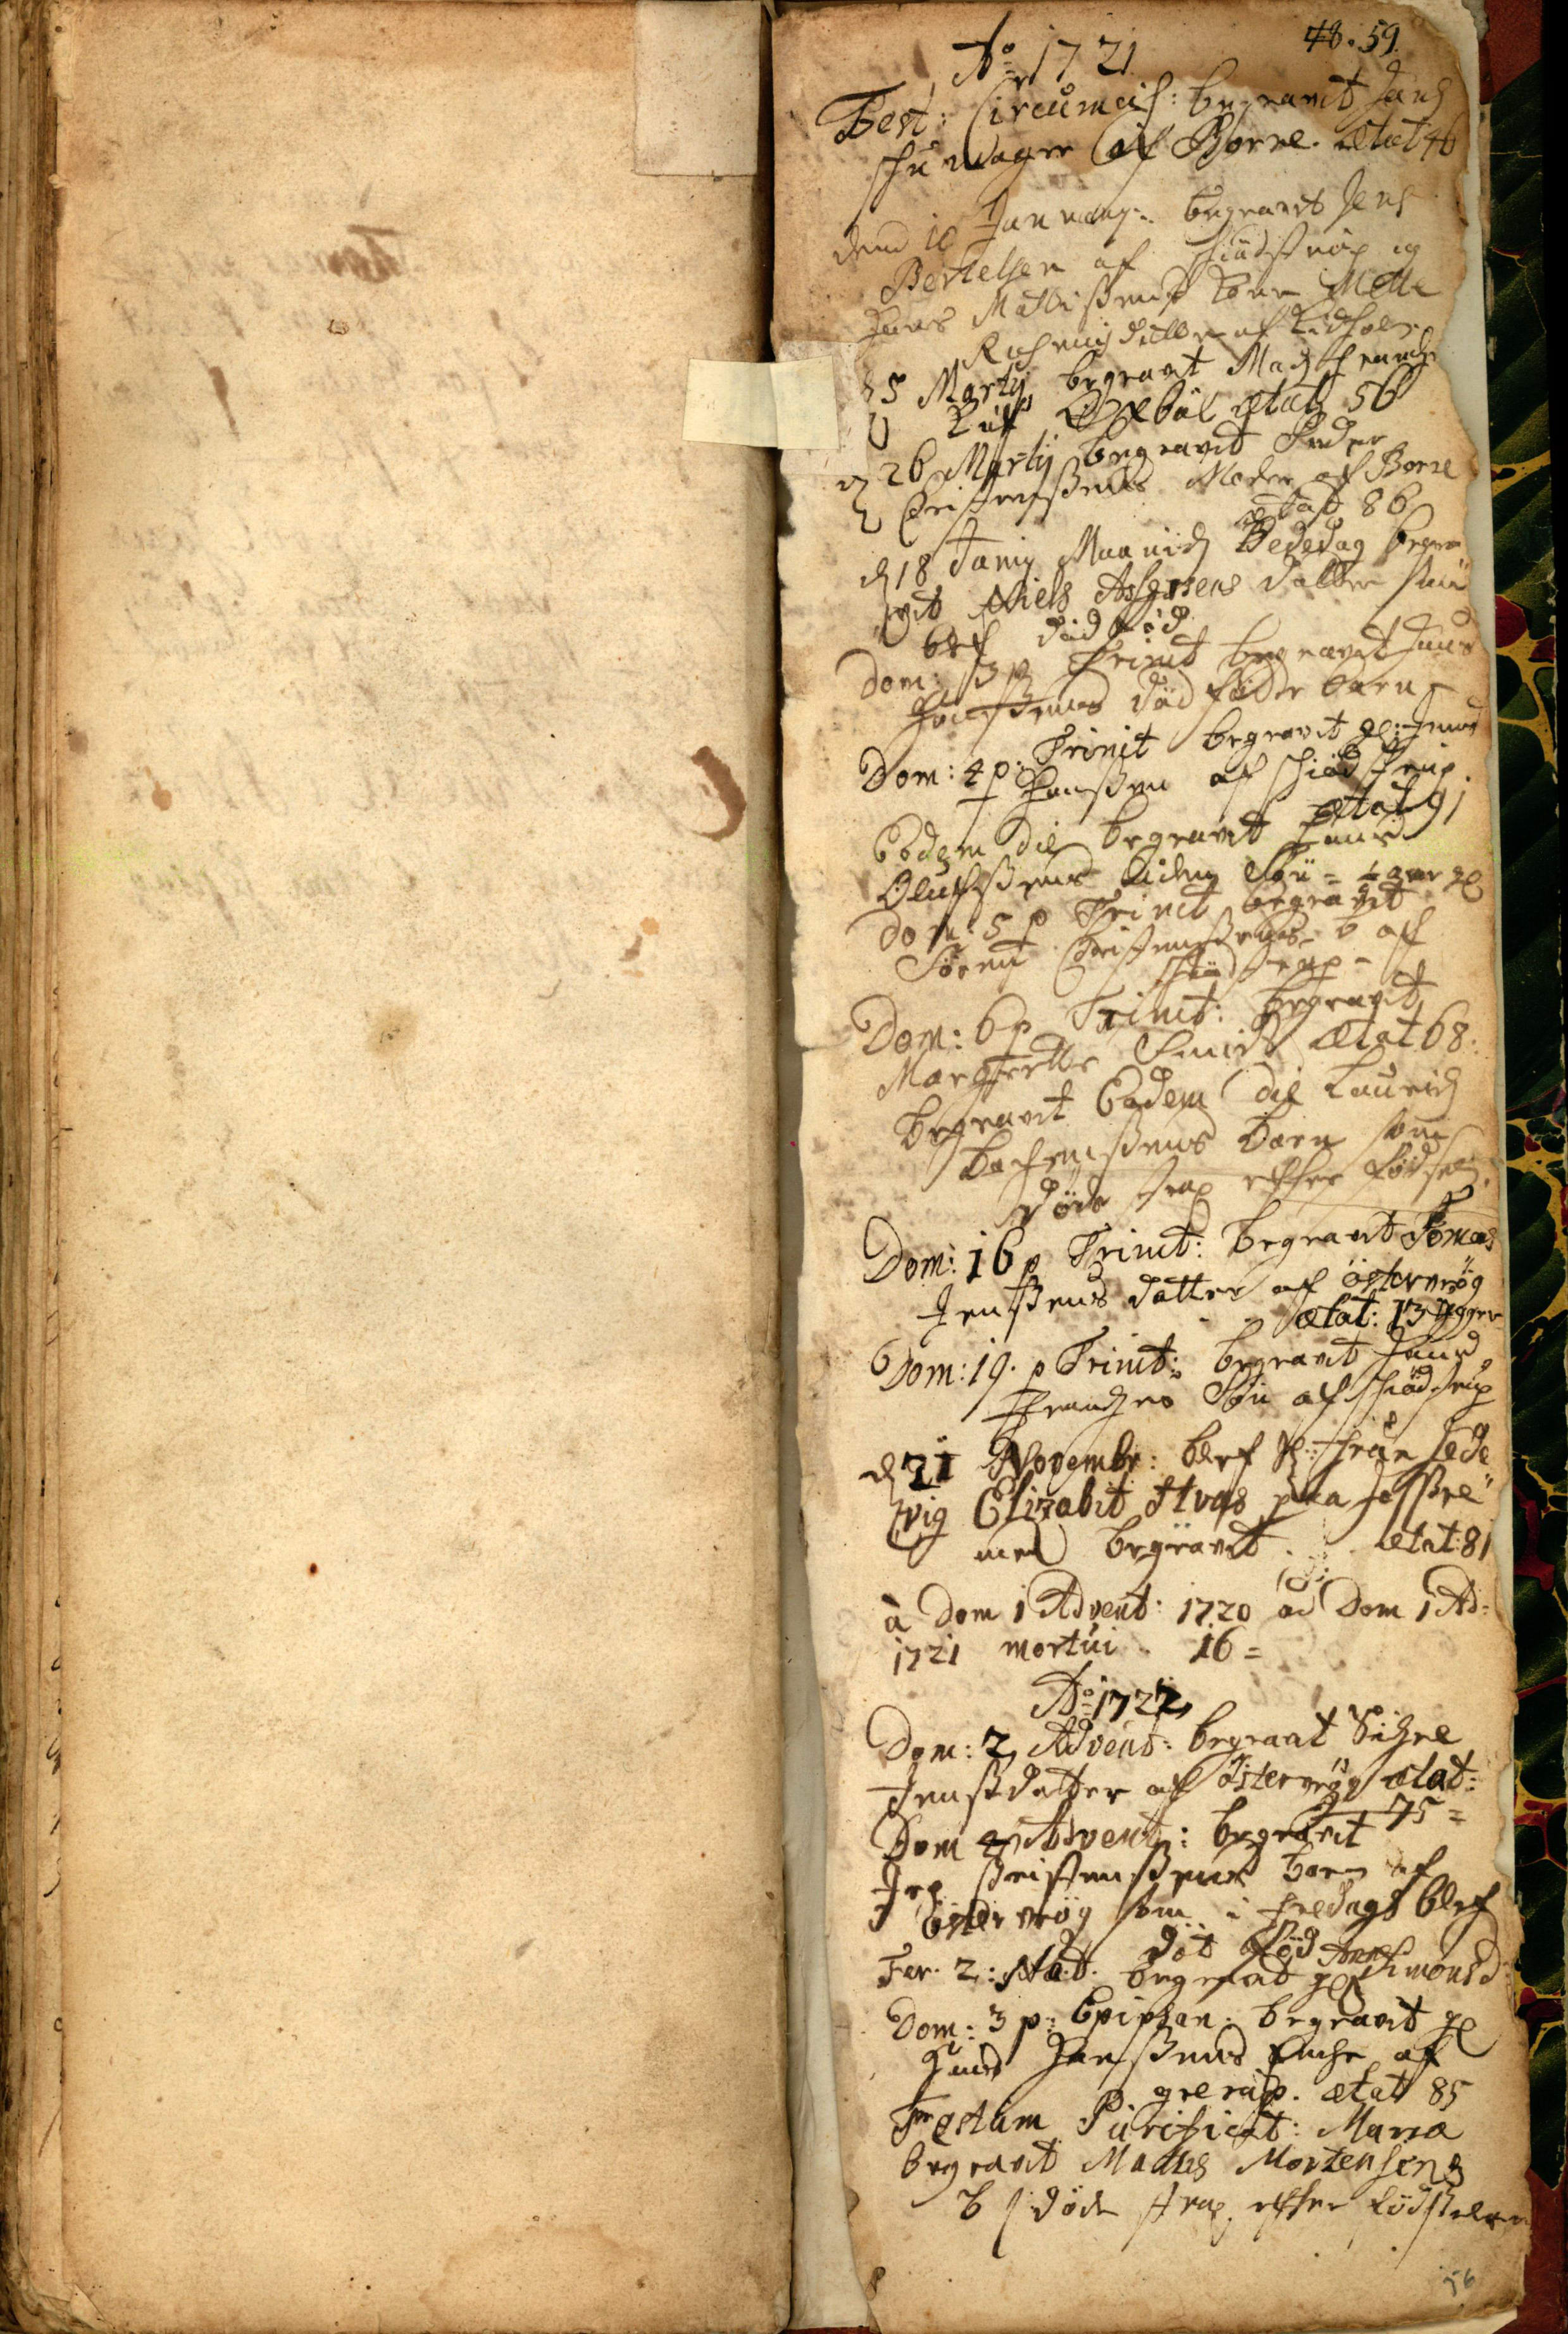48. 59
56
Ao 1721.
Fes :.Circumcis: begravet Hans
Schumager af Borre. ætat 46
Dend 10 Januarij: begravet Jens
Bertelsen af Schiødstrup og
Hans Mattisens Kone Mette
Rasmusdatter af Kidholm
D 5 Martij begravet Madz Frandz
U Kudsk## Oxbøl ætat 56
D 26 Martij begravet Peder
Christensens Moder af Borre
ætat 86
D 18 Junii Maandz Bededag begra¬
vet Niels Assersens Datter som
blef død fød
Dom: 3 p Trinit begravet Hans
Hansens død fødde Barn
Dom: 4 p: Trinit begravet gl:  Jens
Hansen af Schiødstrup
ætat 91
Eodem Die begravet Hans
Olufsens liden Søn= ¼ aar gl
Dom: 5 p Trinit begravet
Søren Christensens B af
Schiødstrup-
Dom: 6 p Trinit: begravet
Margrette Smids ætat 68:
Begravet Eodem Die Lauridz
Bachensens Barn som
døde strax efter Fødselen
Dom:16 p Trinit: begravet Tomas
Jensens Datter af Østervrøg
ætat: 13 Ugger
Dom: 19. p Trinit: begravet Hans
Frandzens Søn af Schiødstrup
D 21 Novembr: blef Sl: Frue Hede¬
vig Elizabet Hvas paa Hessel¬
med begravet ætat: 81
à Dom 1 Advent: 1720 ad Dom 1 Ad:
1721 mortui 16=
Ao 1722
Dom: 2 Advent: begravet Sidzel
Jensdatter af Østervrøg ætat:
75
Dom 4 Advent: begravet
Jep Christensens Barn af
Østervrøg som i Fredags blef
døt fød Anne
Simonsd
Fer. 2: Nat. begravet gl
Dom: 3 p: Epiphan: begravet gl
Hans Hansens Enche af
Grerup ætat 85
Festum Purificat: Mariæ
begravet Mattis Mortensens
B s døde strax efter Fødselen
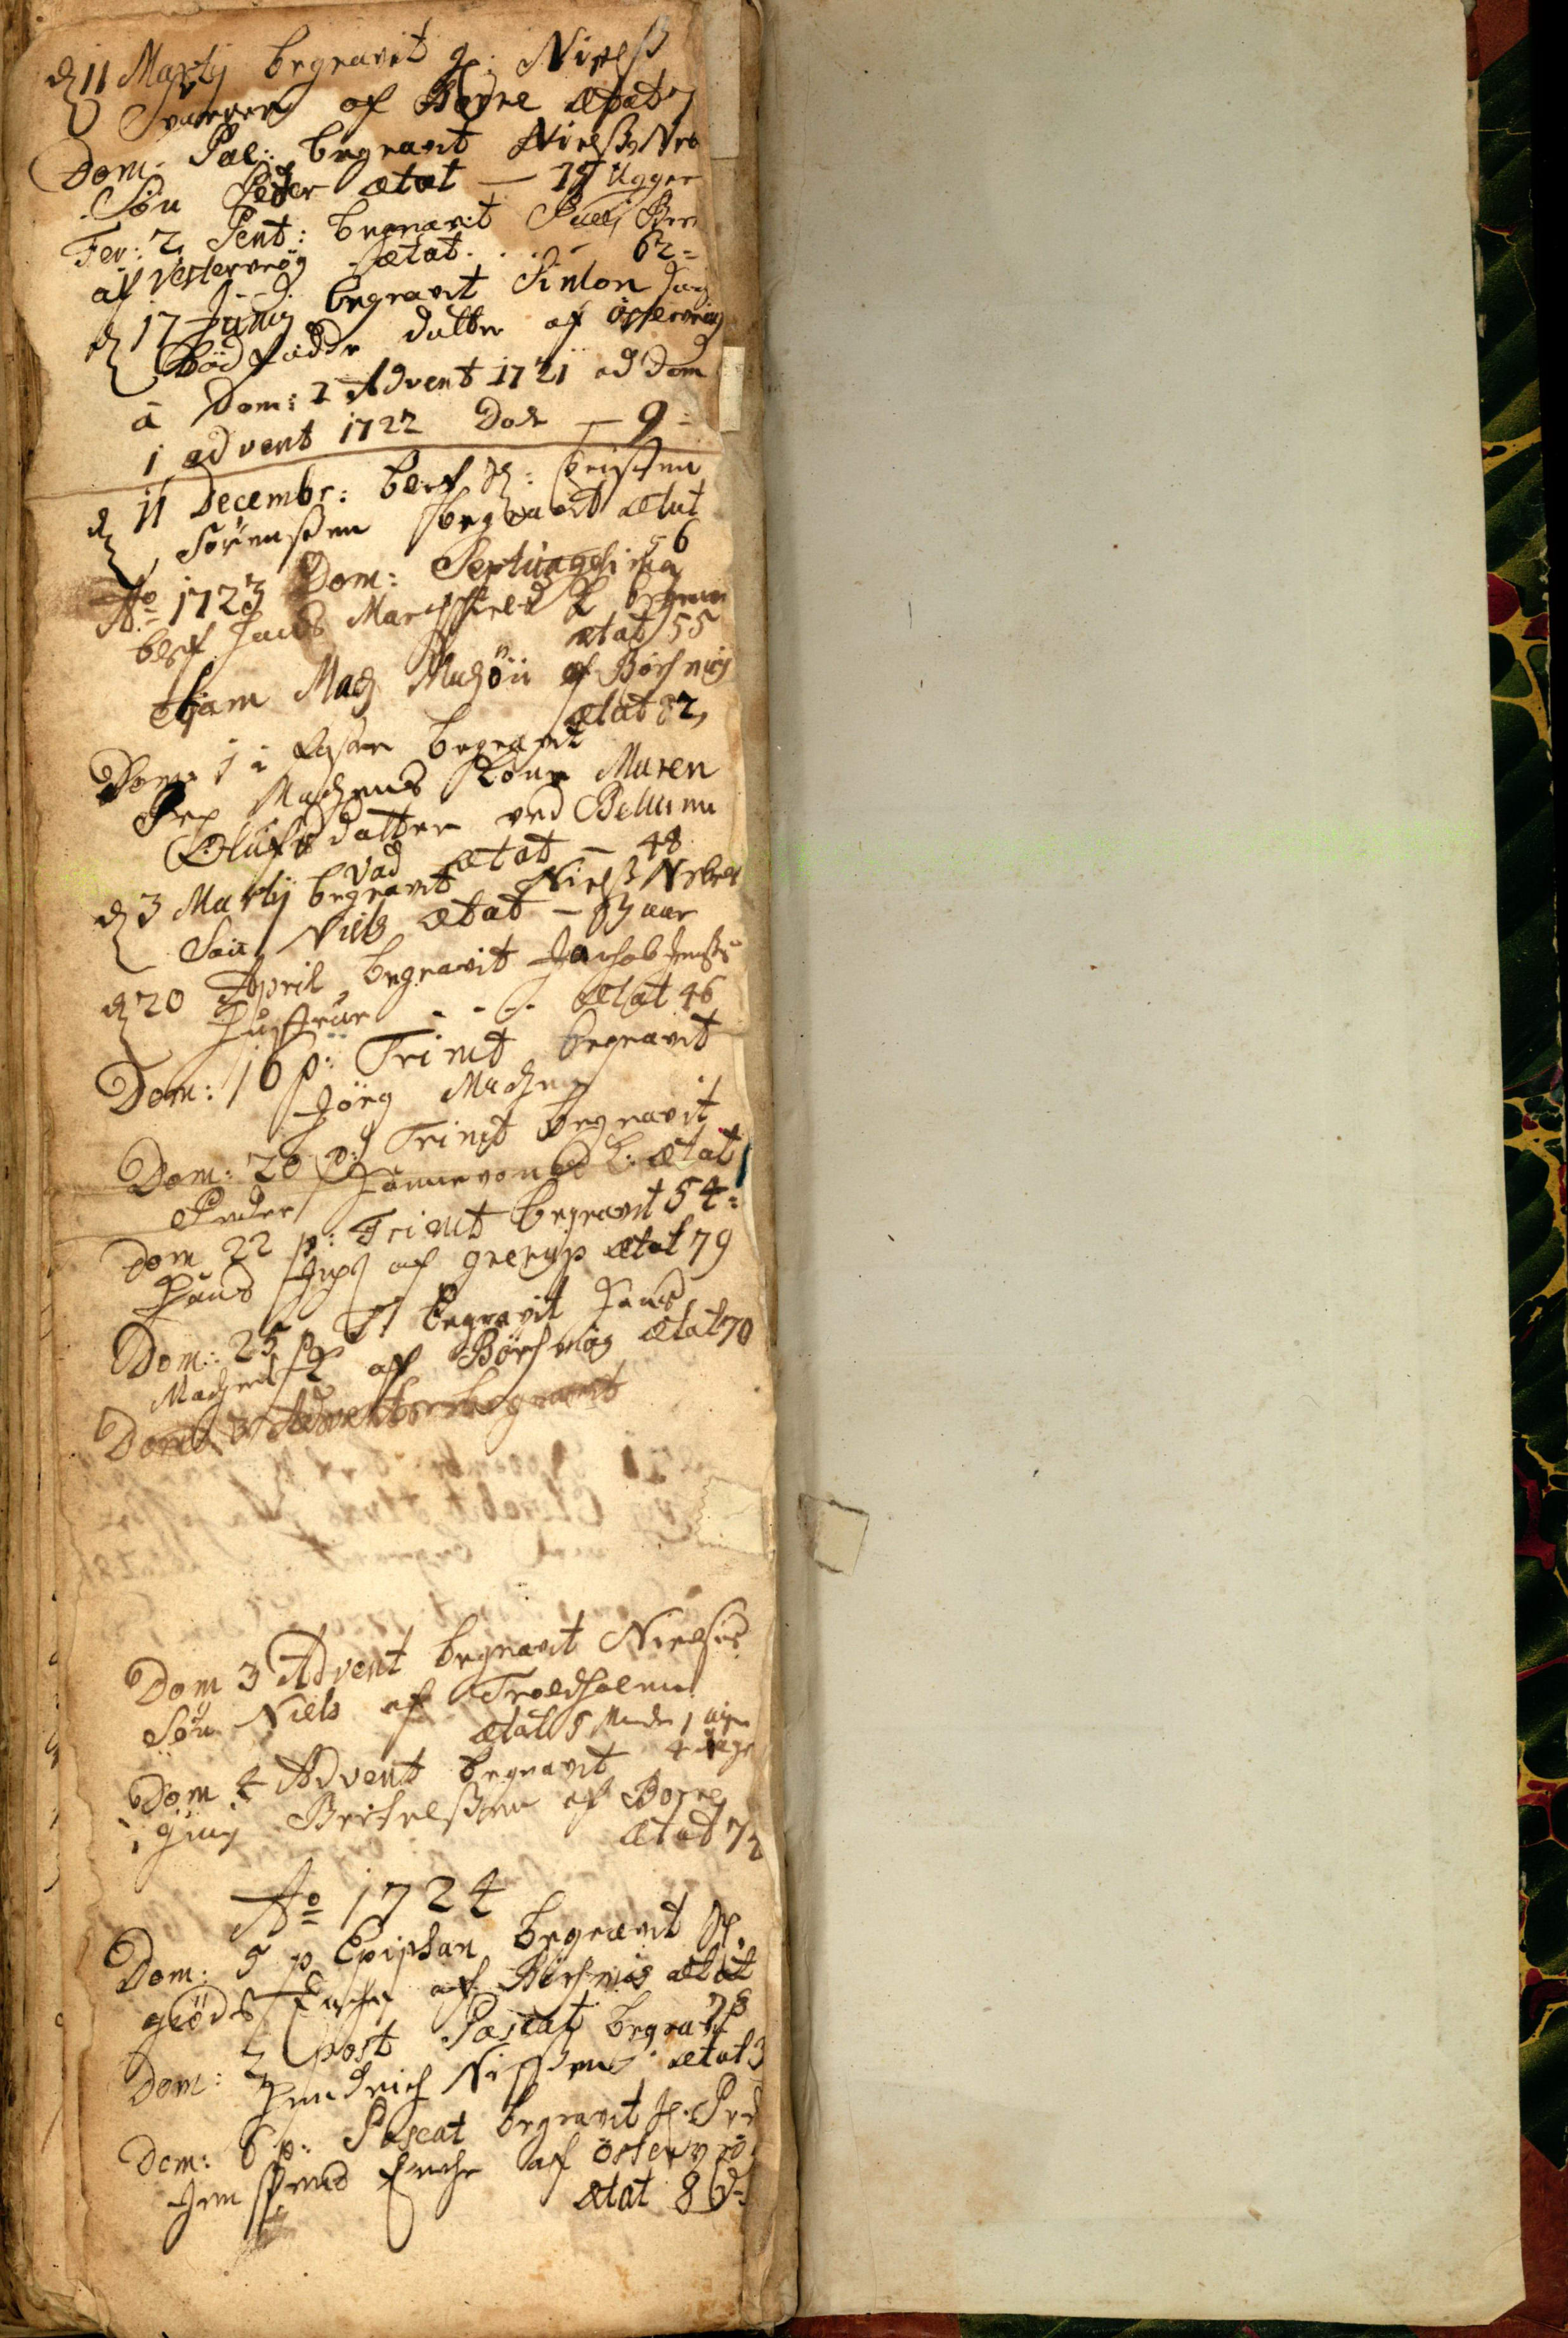D 11 Martij begravet gl: Niels
Svarrer af Borre ætat 7
Dom: Pal: begravet Niels Neb
Søn Peder ætat 15 Ugger
Fer: 2 Pent: begravet Pallj Ber
af Vestervrøg ætat.... 62=
D 17 Junij begravet Simon Hans
død fødde Datter af Østervrøg
à Dom: 1 Advent 1721 ad Dom
1 Advent 1722 Dode 9=
D 11 Decembr blef Sl: Christen
Sørensen begravet ætat
56
Ao 1723 Dom: Septuagesima
blef Hans Marchschiels K begrav
ætat 55
Etiam## Madz Madzøn af Børsmøs
ætat 82,
Dom: 1 i Faste begravet
Jep Madzens Kone Maren
Olufsdatter ved Bellum
vad ætat 48
D 3 Martij begravet Niels Nebels
Søn Niels ætat- 3 aar
D 20 April begravet Jachob Jens
Hustrue.... ætat 46
Dom: 16 p: Trinit begravet
Jørg Madzen
Dom: 20 p: Trinit begravet
Peder Hannevangs K: ætat
Dom 22 p: Trinit begravet 54:
Hans Jeps af Grerup ætat 79
Dom: 25 p T: begravet Hans
Madzens K af Børsmøs ætat 70
Dom: 3 Advent begravet
Dom 3 Advent begravet Nielsis
Søn Niels af Troldholm
ætat 5 Mens 1 uge
4 dage
Dom 4 Advent begravet
Gunj Bertelsen af Borre
ætat 72
Ao 1724
Dom: 5 p Epiphan begravet Sl.
Giødes Enche af Børsmøs ætat
78
Dom: 2 post Pascat begravet
Hensdrich Nissen ætat 3
Dom: 6 p: Pascat begravet Sl. Ped
Jensens Enche af Østervrøg
ætat 86=